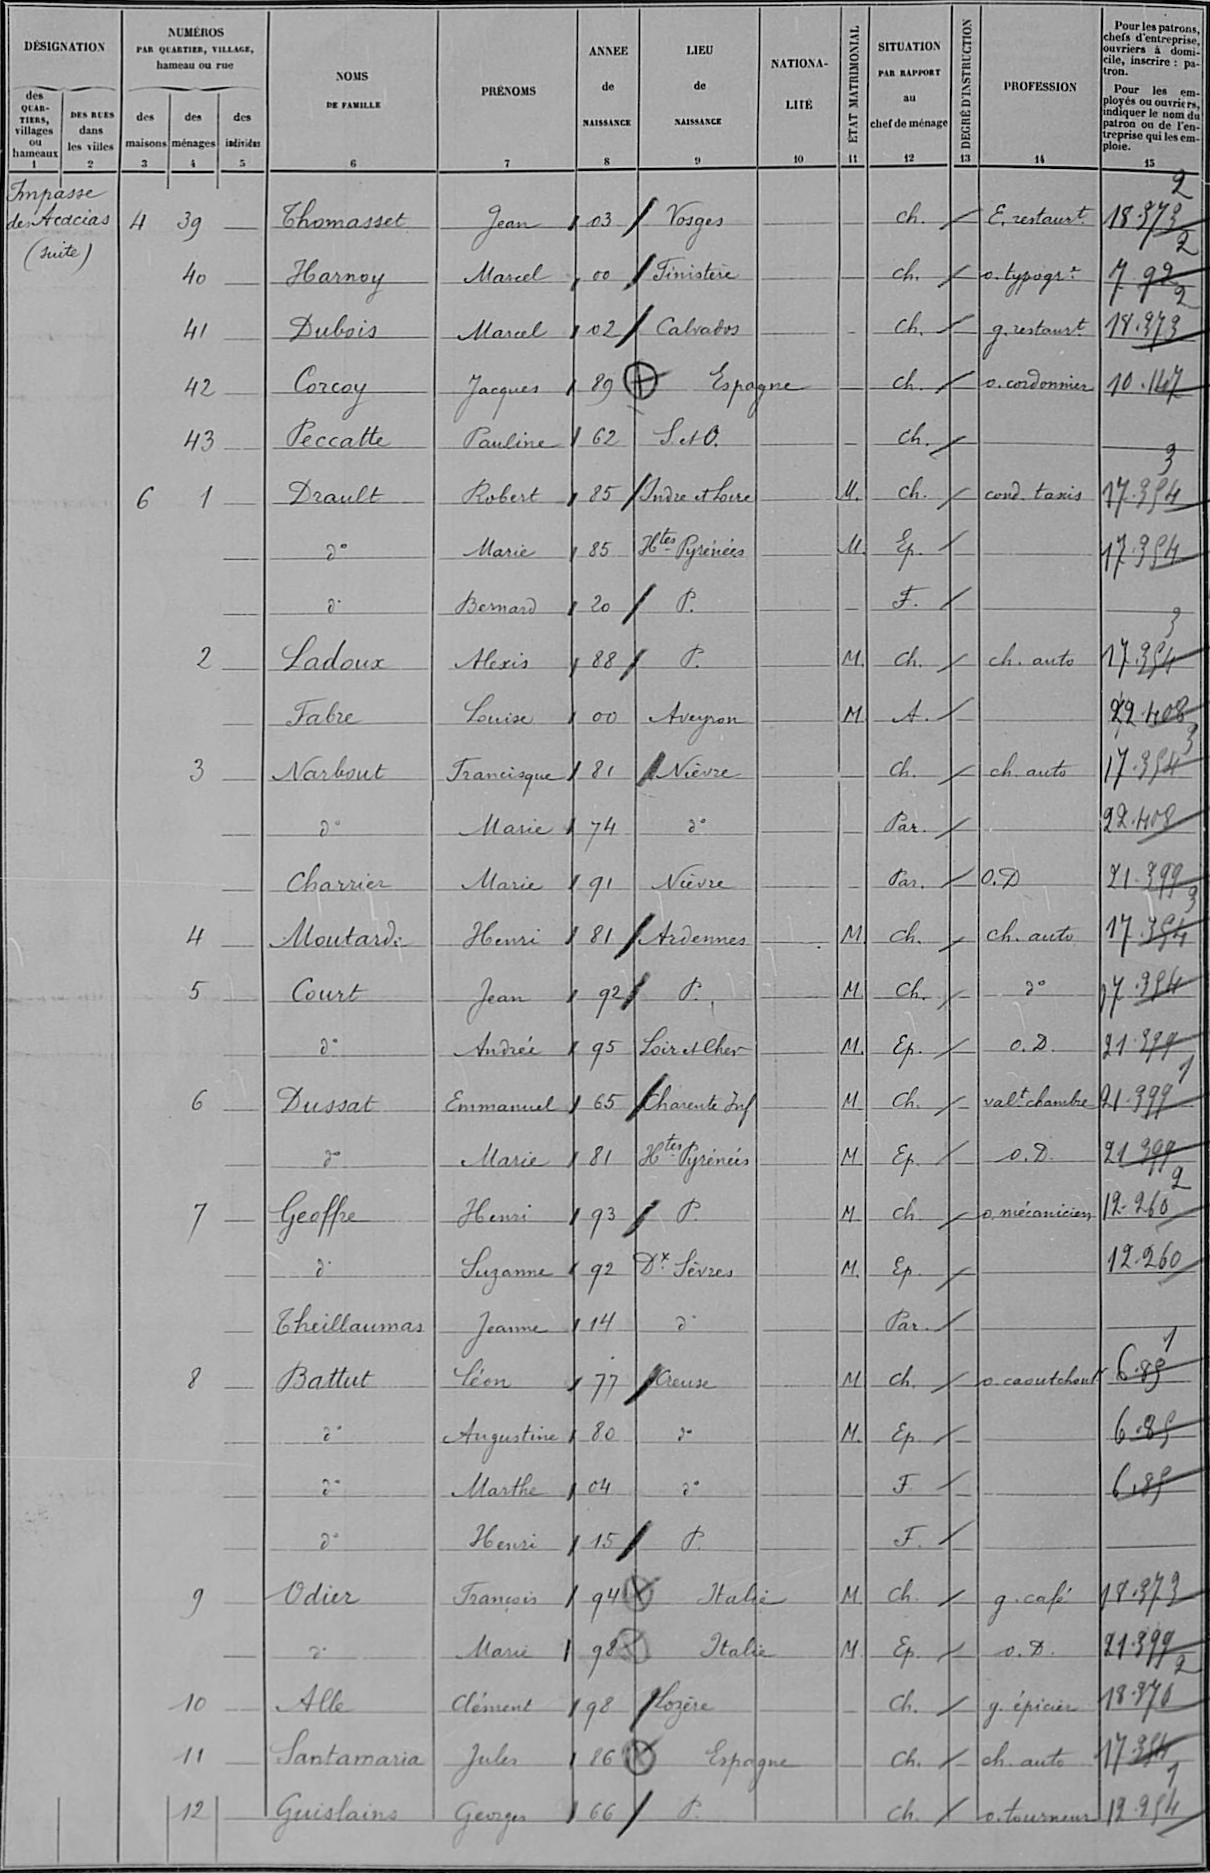Thomasset/Jean/03/Vosges/¤/¤/ch /¤/E restaurt/183732
Harnoy/Marcel/00/Finistère/¤/¤/ch /¤/o typogre/7922
Dubois/Marcel/02/Calvados/¤/¤/ch /¤/g restaurt/18 373
Corcoy/Jacques/89/Espagne/¤/¤/ch /¤/o coordonnier/10 147
Peccatte/Pauline/62/S et O /¤/¤/ch /¤/¤/¤
Drault/Robert/25/Indre et Loire/¤/M /ch /¤/cond taxis/17354
d°/Marie/85/Htes Pyrenees/¤/M /Ep /¤/¤/17354
d°/Bernard/20/P /¤/¤/F /¤/¤/¤
Ladoux/Alexis/88/P /¤/M /ch /¤/ch auto/17354
Fabre/Louise/00/Aveyron/¤/M/A /¤/¤/22408
Narbout/Francisque/81/Nièvre/¤/¤/Ch /¤/ch auto/173543
d°/Marie/74/d°/¤/¤/Par /¤/¤/22408
Charrier/Marie/91/Nièvre/¤/¤/Par /¤/O D/213993
Moutard/Henri/81/Ardennes/¤/M/ch /¤/ch auto/17354
Court/Jean/92/P /¤/M/Ch /¤/d°/17354
d°/Andrée/95/Loir et Cher/¤/M/Ep /¤/O D/213991
Dussat/Emmanuel/65/Charente Inf/¤/M/Ch/¤/valt chambre/21 3991
d°/Marie/81/Htes Pyrénées/¤/M/Ep/¤/O D /21 3992
Geoffre/Henri/93/P /¤/M/ch/¤/o mécanicien/12 260
d°/Suzanne/92/Dx Sèvres/¤/M /Ep /¤/¤/12 260
Theillaumas/Jeanne/14/d°/¤/¤/Par /¤/¤/¤
Battut/Léon/77/Creuse/¤/M/Ch /¤/o caoutchoutr/6 85
d°/Augustine/80/d°/¤/M /Ep/¤/¤/6 85
d°/Marthe/04/d°/¤/¤/F/¤/¤/6 85
d°/Henri/15/P /¤/¤/F/¤/¤/¤
Odier/François/94/Italie/¤/M/Ch /¤/g café/18 373
d°/Marie/98/Italie/¤/M/Ep/¤/O D /213992
Alle/Clément/98/Lozère/¤/¤/Ch /¤/g épicier/18370
Santamaria/Jules/86/Espagne/¤/¤/Ch /¤/ch auto/173541
Guislains/Georges/66/d°/¤/¤/Ch /¤/o tourneur/12 254
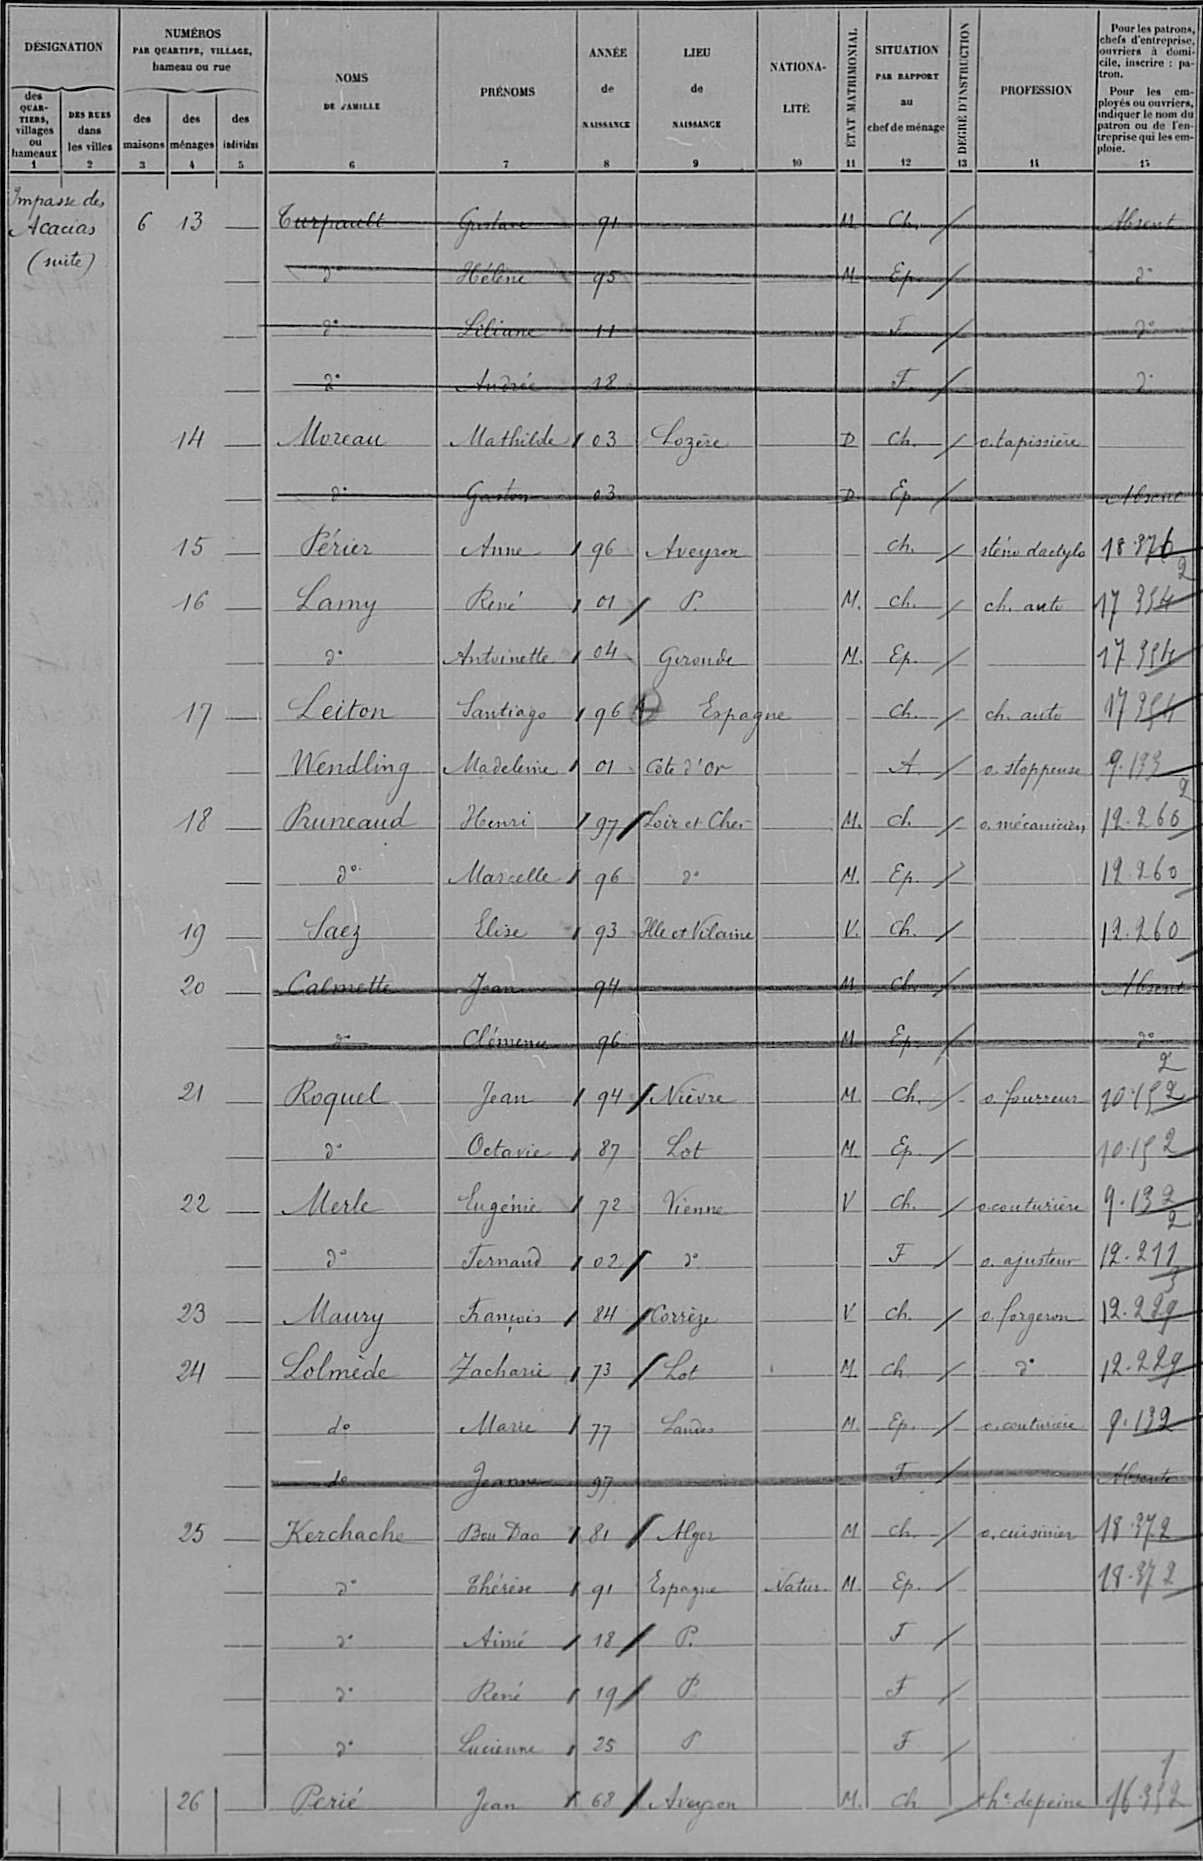Turpault/Gustave/91/¤/¤/M/Ch/¤/¤/Absent
d°/Hélène/95/¤/¤/M/Ep/¤/¤/d°
d°/Liliane/11/¤/¤/¤/F/¤/¤/d°
d°/Andrée/18/¤/¤/¤/F /¤/¤/d°
Moreau/Mathilde/03/Lozère/¤/D/ch /¤/o tapissière/¤
d°/Gaston/03/¤/¤/D/Ep/¤/¤/Absent
Périer/Anne/96/Aveyron/¤/¤/ch /¤/sténo dactylo/183762
Lamy/René/01/P /¤/M/ch /¤/ch auto/173542
d°/Antoinette/04/Gironde/¤/M/Ep /¤/¤/17354
Leiton/Santiago/96/Espagne/¤/¤/Ch /¤/ch auto/17354
Wendling/Madeleine/01/Cote d'Or/¤/¤/A/¤/o stoppeuse/9133
Pruneaud/Henri/97/Loir et Cher/¤/M/ch/¤/o mécanicien/122602
d°/Marcelle/96/d°/¤/M/Ep /¤/¤/12260
Saez/Elise/93/Ile et Vilaine/¤/V /ch /¤/¤/12260
Calmette/Jean/94/¤/¤/M/ch/¤/¤/Absent
d°/Clémence/96/¤/¤/M/Ep/¤/¤/d°2
Roquel/Jean/94/Nièvre/¤/M/ch /¤/o fourreur/10752
d°/Octavie/87/Lot/¤/M/Ep/¤/¤/10152
Merle/Eugénie/72/Vienne/¤/V/Ch /¤/o couturière/9 1322
d°/Fernand/02/d°/¤/¤/F/¤/o ajusteur/12 2113
Maury/François/84/Corrèze/¤/V/Ch/¤/o forgeron/12229
Lolmède/Zacharie/73/Lot/¤/M/ch /¤/d°/12 229
d°/Marie/77/Landes/¤/M /Ep /¤/o couturière/9 132
d°/Jeanne/97/¤/¤/¤/F/¤/¤/Absente
Kerchache/Bou Dao/81/Alger/¤/M/ch/¤/o cuisinier/18372
d°/Thérèse/91/Espagne/Natur/M /Ep /¤/¤/18372
d°/Aimé/18/P /¤/¤/F/¤/¤/¤
d°/René/19/P/¤/¤/F/¤/¤/¤
d°/Lucienne/25/P/¤/¤/F/¤/¤/¤
Perié/Jean/68/Aveyron/¤/M/Ch/¤/he de peine/163521